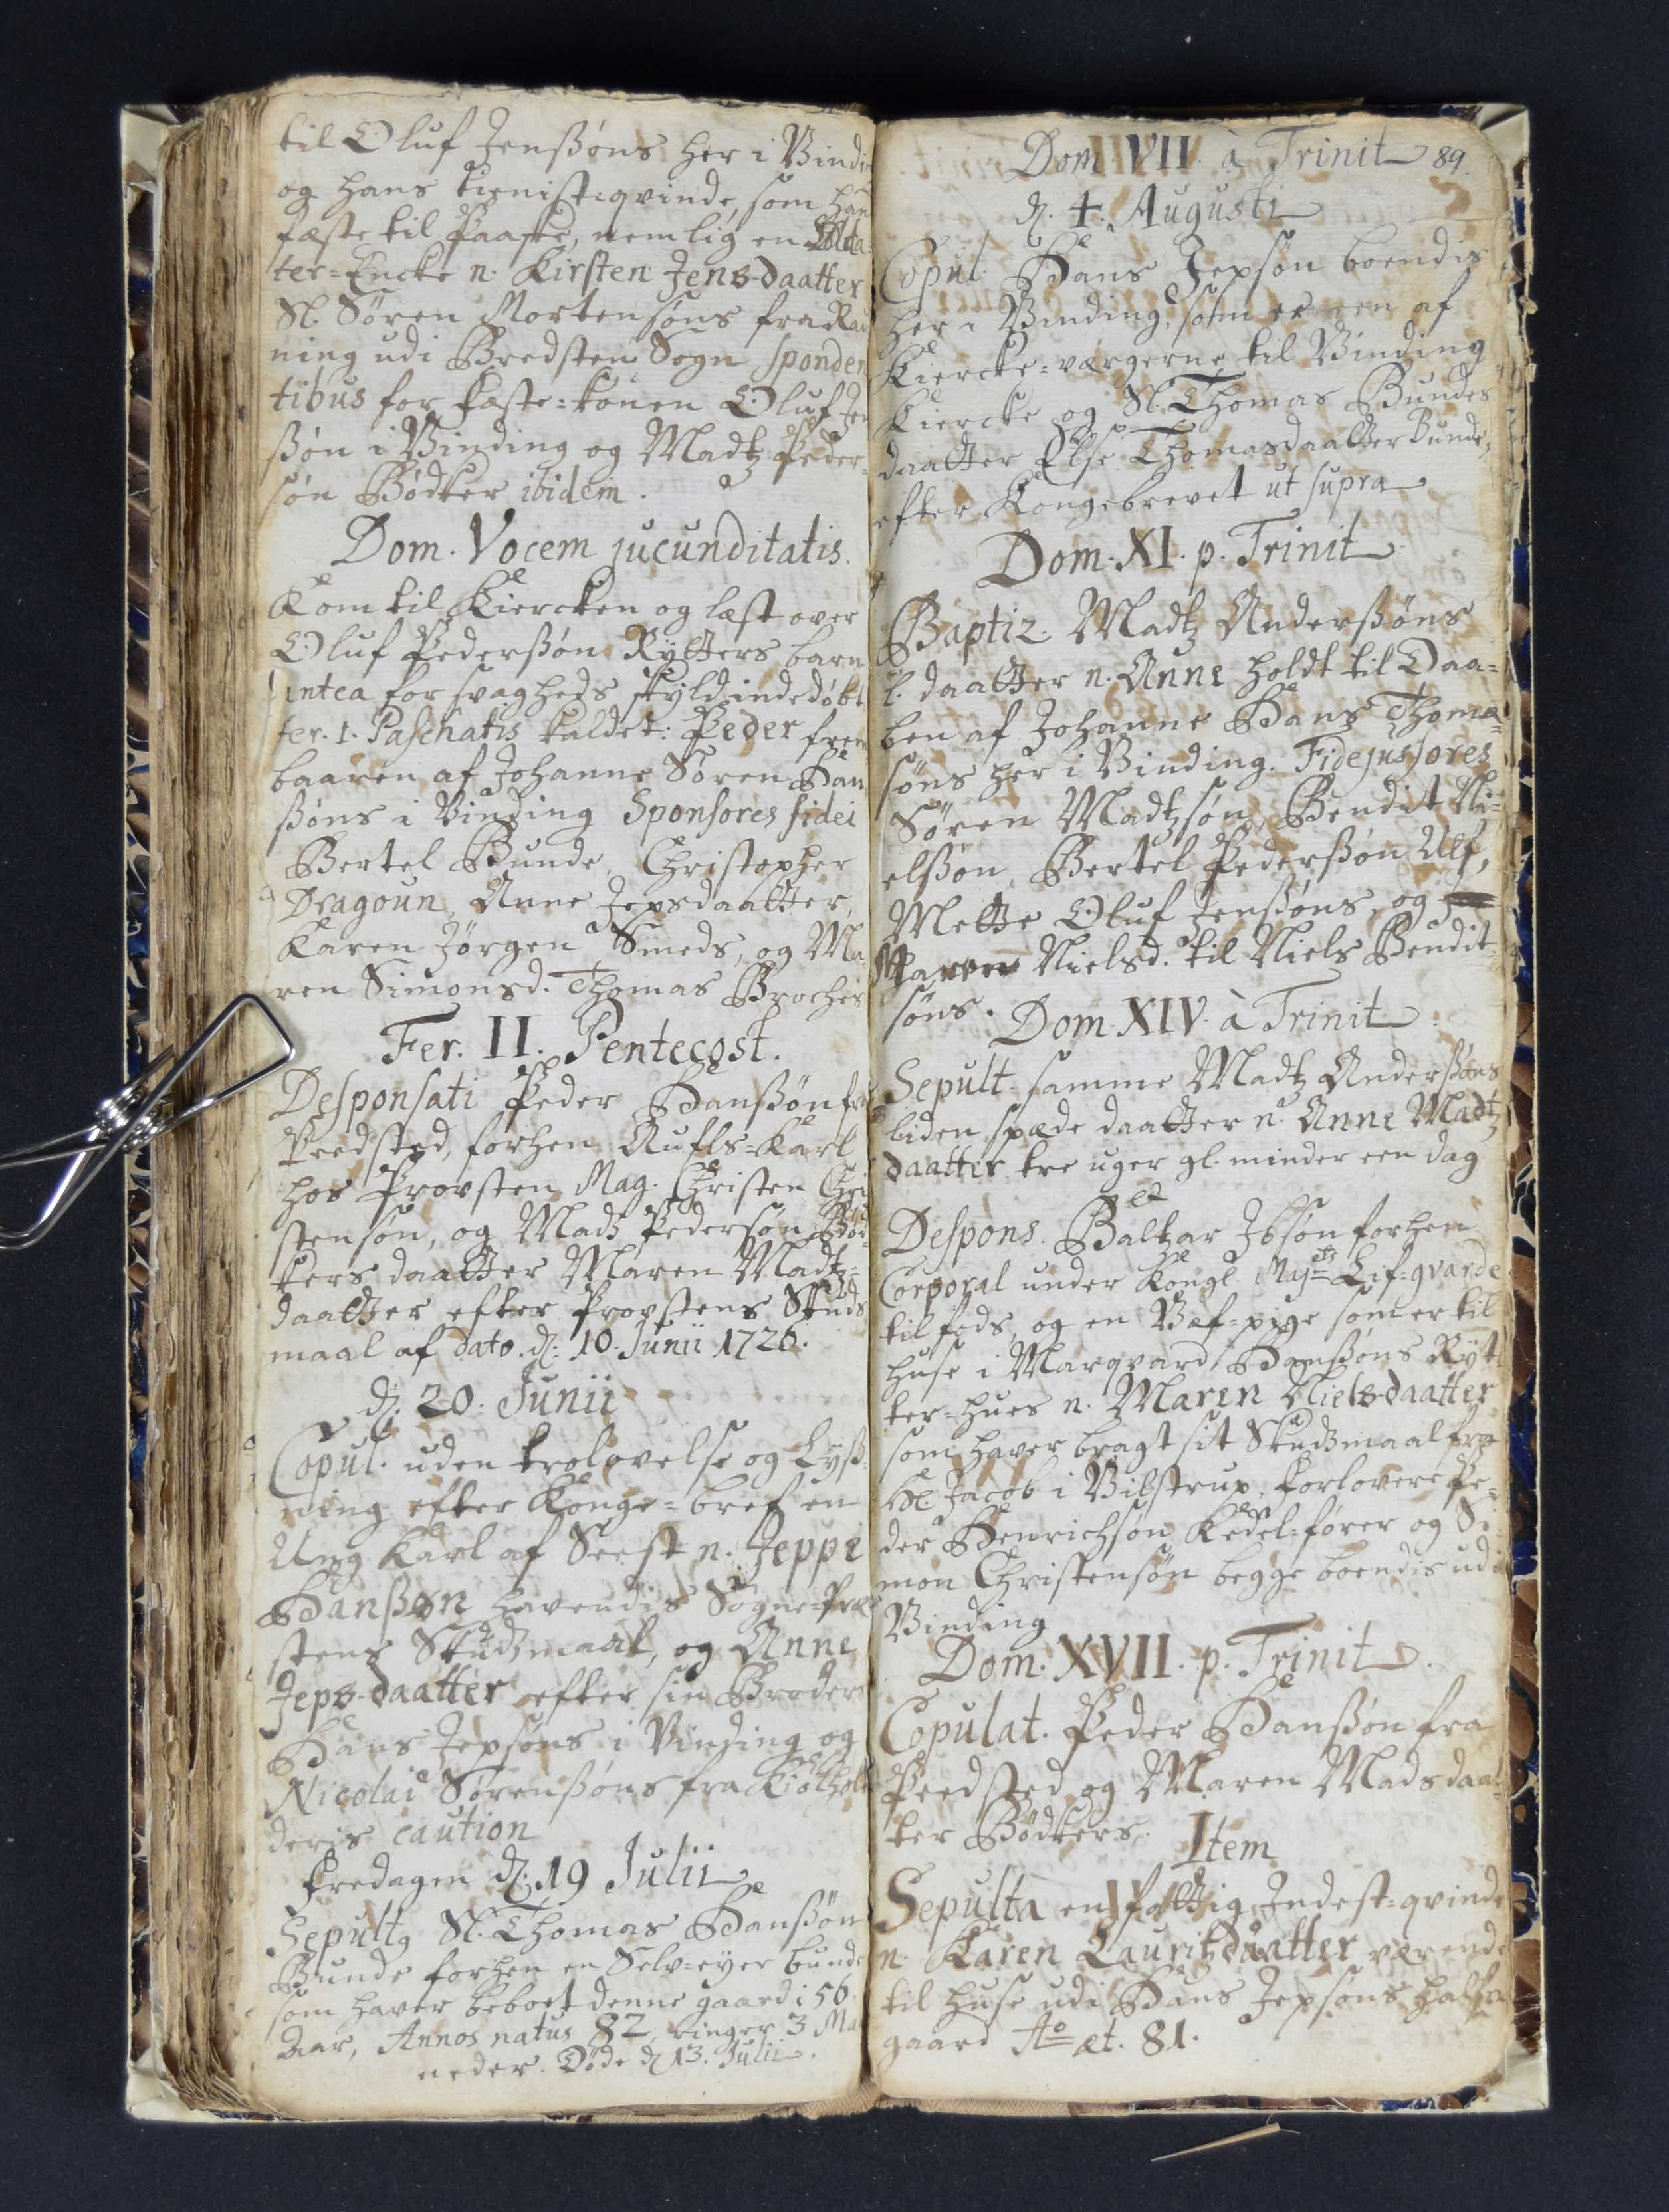til Oluf Jenssøns her i Vindin 
og hans tienisteqvinde, som han 
fæste til Paaske, nemlig en Solda¬
ter=Encke n. Kirsten Jens-daatter
Sl. Søren Mortensøns fra Rau
ning udi Bredsten Sogn Sponden
tibus for Fæste=konen Oluf Jen
ssøn i Vinding og Madtz Peder¬
søn Bødker ibidem.
Dom. Vocem jucunditatis
Kom til Kiercken og læst over
Oluf Pederssøns Rytters barn
ntea for svagheds skyld indedøbt
fer. I. Paschatis kaldet: Peder, frem¬
baaren af Johanne Søren Han
ssøns i Vinding Sponsores fidei
Bertel Bunde, Christopher
Dragoun, Anna Jepsdaatter
Karen Jørgen Smeds, og Ma¬
ren Simonsd. Thomas Brochis
Fer. II. Pentecost.
Desponsati. Peder Hanssøn fra
Piedsted, forhen Aufls=Karl
hos Provsten Mag. Christen Chri
stensøn, og Madz Pedersøn Bød¬
kers daatter Maren Madtz¬
daatter efter Provstens Skuds
maal af dato d: 10. Junii 1726
d. 20. Junii
Copul. uden trolovelse og Lyss¬
ning efter Konge=bref en
Ung Karl af Seest n. Jeppe
Hanssøn havendis Sogne=Præ
stens Skudsmaal, og Anne 
Jeps-daatter efter sin Broder
Hans Jepsøns i Vinding og 
Nicolai Sørenssøns fra Kiølholt
deris caution
Fredagen d. 19 Julii
Sepultq Sl. Thomas Hanssøn
Bunde forhen en Selv=eyer bunde
som haver beboet denne gaard i 56
Aar, Annos natus 82 ringere 3 Ma
aneder, Døde d 13. Julii.
89.
Dom. VII. a Trinit
d. 4. Augusti.
Copul. Hans Jepsøn boendis
her i Vinding, som er den ene af
Kiercke=værgerne til Vinding
Kiercke og Sl. Thomas Bundes
daatter Else Thomasdaatter Bunde
efter Kongebrevet ut supra
Dom. XI. p. Trinit
Baptiz. Madtz Anderssøns
l. daatter n. Anne holdt til Daa¬
ben af Johanne Hans Thomæ¬
søns her i Vinding. Fidejussores
Søren Madtzsøn, Bendit Ni¬
elssøn, Bertel Pederssøn Ulf
Mette Oluf Jenssøns, og
Maren Nielsd. til Niels Bendit¬
søns.
Dom. XIV. a Trinit
Sepult. samme Madtz Anderssøns
liden spæde daatter n. Anna Madtz
daatter tre uger gl. minder een dag
et
Despons. Baltazar Ibsøn forhen
Corporal under Kongl. Mayets Lif=qvarde
til fods og en Væf=pige som er til
huse i Marqvard Hanssøns Ryt
ter=hues n. Maren Niels-daatter,
som haver bragt sit Skudsmaal fra
Hl Jacob i Vilstrup, Forlovere Pe¬
der Henrichsøn Kedel=fører og Si
mon Christensøn begge boendis udi
Vinding
Dom. XVII. p. Trinit
Copulat. Peder Hanssøn fra
Peedsted og Maren Madsdaat¬
ter Bødkers.
Item
Sepulta en fattig Indest=qvinde
n. Karen Lauridtzdaatter værende
til huse udi Hans Jepsøns half##
gaard Ao æt. 81.